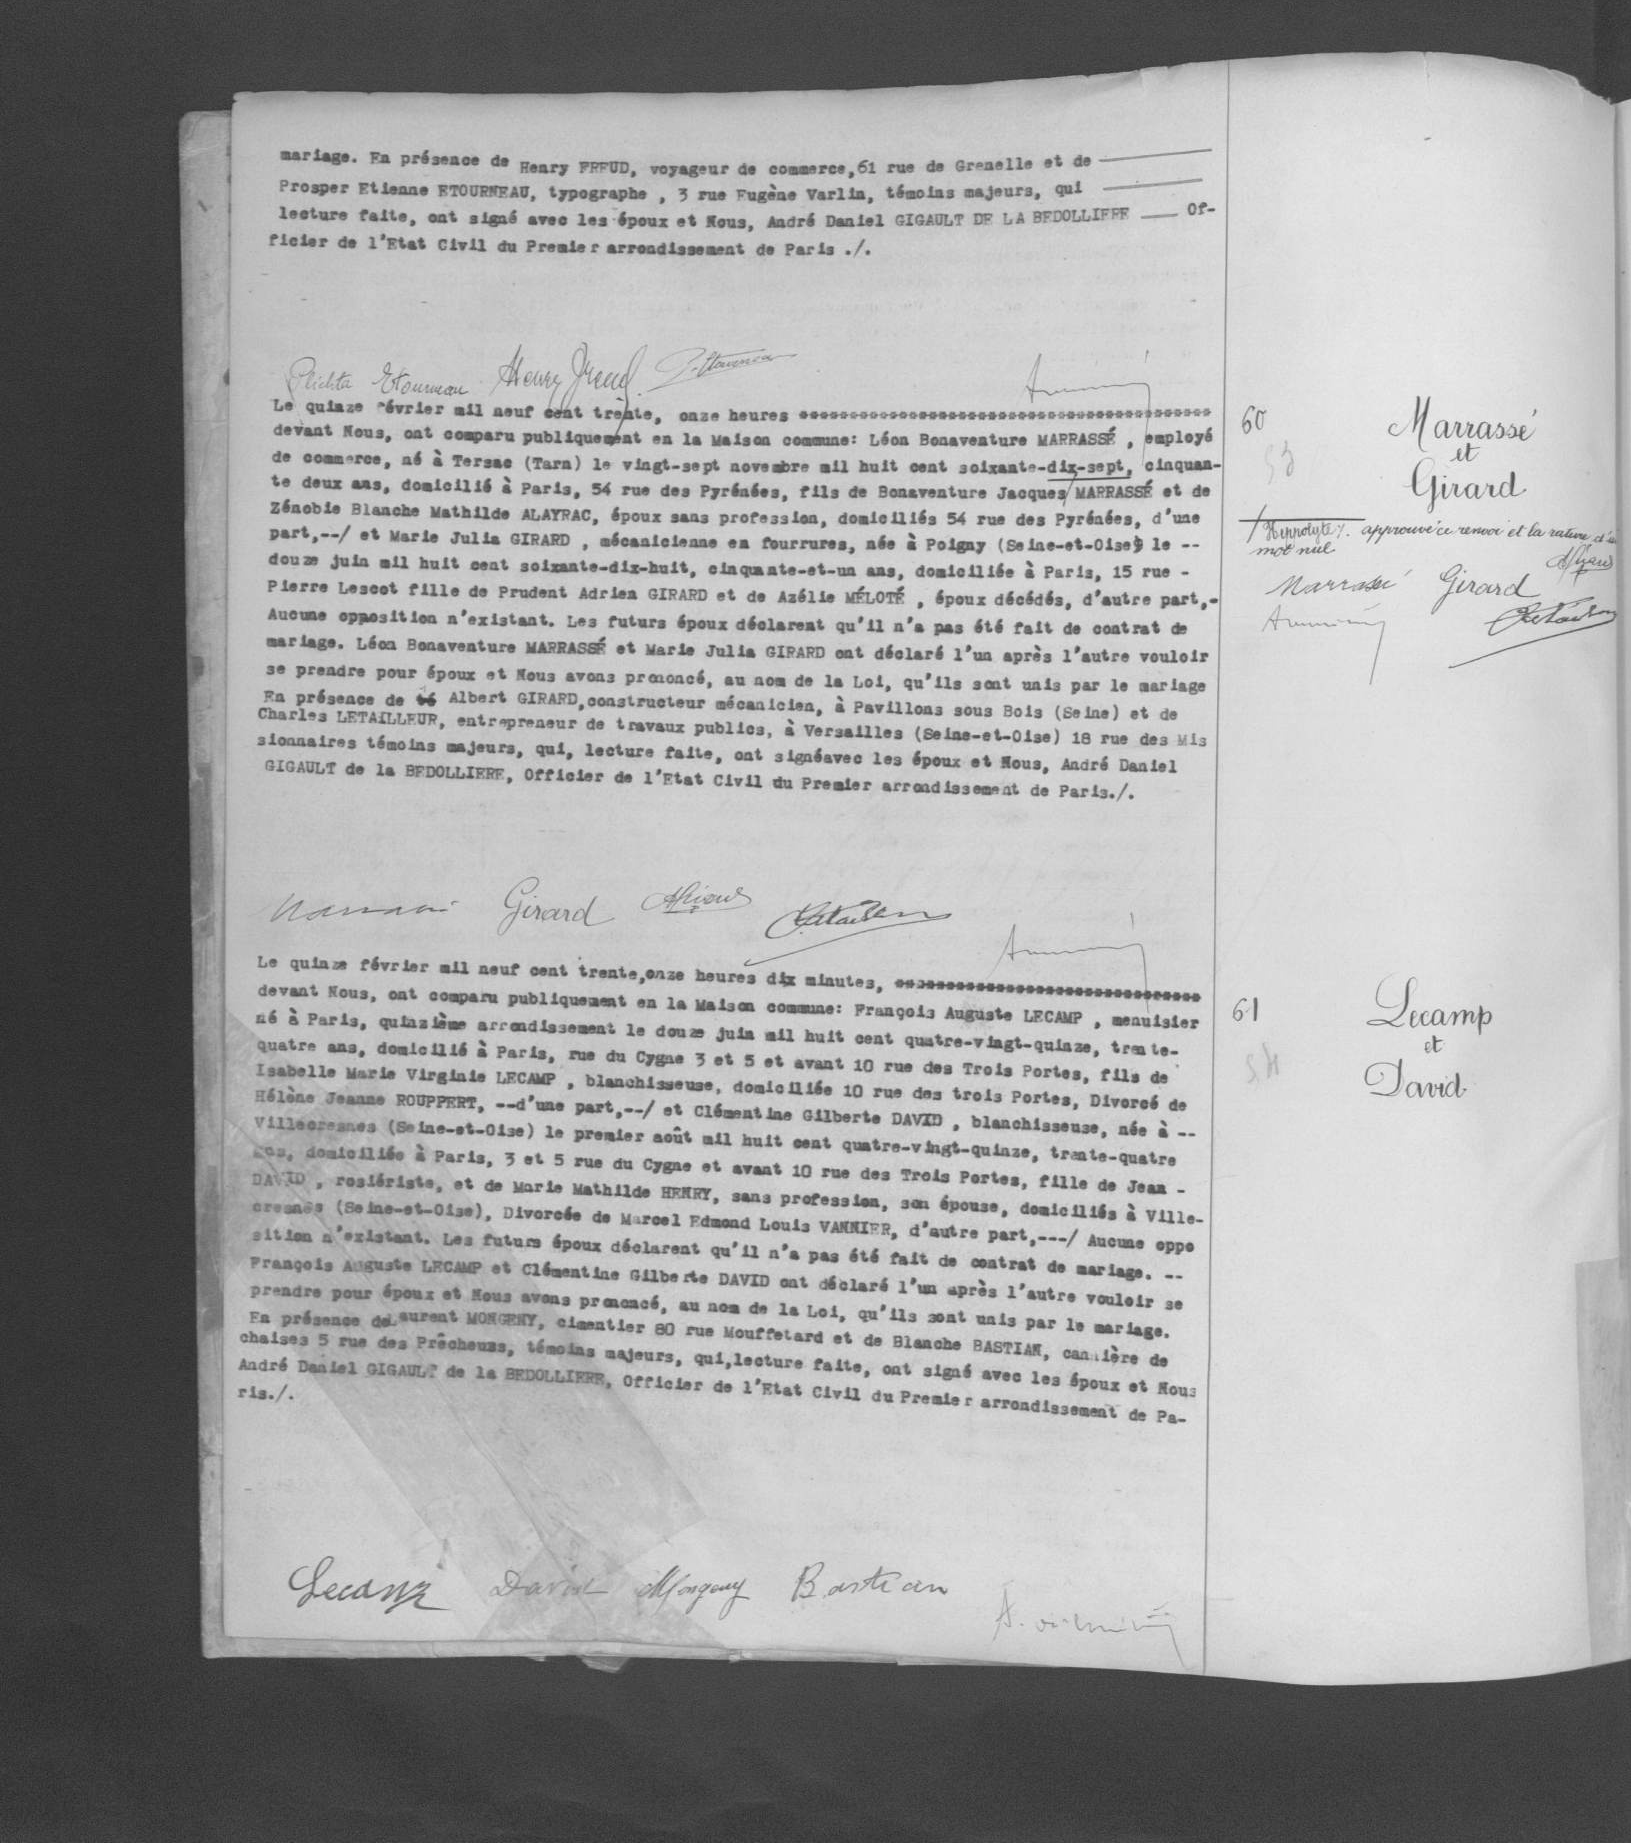mariage. En présence de Henry FREUD, voyageur de commerce, 61 rue de Grenelle et de -
Prosper Etienne ETOURNEAU, typographe, 3 rue Eugène Varlin, témoins majeurs, qui -
lecture faite, ont signé avec les époux et Nous, Anré Daniel GIGAULT DE LA BEDOLLIERE -Of-
ficier de l'Etat Civil du Premier arrondissement de Paris ./.
Le quinze février mil neuf cent trente, onze heures ****
devant Nous, ont comparu publiquement en la Maison commune: Léon Bonaventure MARRASE, empoyé
de commerce, né à Tersac (Tarn) le vingt-sept novembre mil huit cent soixante-dix-sept, cinquan-
te deux ans, domicilié à Paris, 54 rue des Pyrénées, fils de Bonaventure Jacques MARRASE et de
Zénobis Blanche Mathilde ALAYRAC, époux sans profession, domiciliés 54 rue des Pyrénées, d'une
part ,-/ et Marie Julia GIRARD, mécanicienne en fourrures, née à Poigny (Seine-et-Oise) le -
douze juin mil huit cent soixante-dix-huit, cinquante-et-un ans, domiciliée à Paris, 15 rue-
Pierre Lescot fille de Prudent Adrien GIRARD et de Azélie MELOTE, époux décédés, d'autre part ,-
Aucune opposition n'existant. Les futurs époux déclarent qu'il n'a pas été fait de contrat de
mariage. Léon Bonaventure MARRASE et Marie Julia GIRARD ont déclaré l'un après l'autre vouloir
se prendre pour époux et Nous avons prononcé, au nom de la Loi, qu'ils sont unis par le mariage
En présence de Albert GIRARD, constructeur mécanicien, à Pavillons sous Bois (Seine) et de
Charles LETAILLEUR, entrepreneur de travaux publics, à Versailles (Seine-et-Oise) 18 rue des Mis
sionnaires témoins majeurs, qui, lecture faite, ont signé avec les époux et Nous, André Daniel
GIGAULT de la BEDOLLIERE, Officier de l'Etat civil du Premier arrondissement de Paris ./.
Le quinze février mil neuf cent trente, onze heures dix minutes, ****
devant Nous, ont comparu publiquement en la Maison commune: François Auguste LECAMP, menuisier
né à Paris, quinzième arrondissement le douze juin mil huit cent quatre-vingt-quinze, trente-
quatre ans, domicilié à Paris, rue du Cygne 3 et 5 et avant 10 rue des Trois Portes, fils de
Isabelle Marie Virginie LECAMP, blanchisseuse, domiciliée 10 rue des trois Portes, Divorcé de
Hélène Jeanne ROUPPERT, -d'une part, -/ et Clémentine Gilberte DAVID, blanchisseuse, née à -
Villecreanes (Seine-et-Oise) le premier août mil huit cent quatre-vingt-quinze, trante-quatre
ans, domiciliée à Paris, 3 et 4 rue du Cygne et avant 10 rue des Trois Portes, fille de Jean
DAVID, rosiériste, et de Marie Mathilde HENRY, sans profession, son épouse, domiciliés à Ville-
cresnes (Seine-et-Oise), Divorcée de Marcel Edmond Louis VANNIER, d'autre part, -/ Aucune oppo
sition n'existant. Les futurs époux déclarent qu'il n'a pas été fait de contrat de mariage .-
François Auguste LECAMP et Clémentine Gilberte DAVID ont déclaré l'un après l'autre vouloir se
prendre pour époux et Nous avons prononcé, au nom de la Loi, qu'ils sont unis par le mariage.
En présence de Laurent MONGENY, cimentier 80 rue Mouffetard et de Blanche BASTIAN, cannière de
chaises 5 rue des Prêcheurs, témoins majeurs, qui, lecture faite, ont signé avec les époux et Nous
Andre Daniel GIGAULT de la BEDOLLIERE, Officier de l'Etat Civil du Premier arrondissement de Pa-
ris ./.
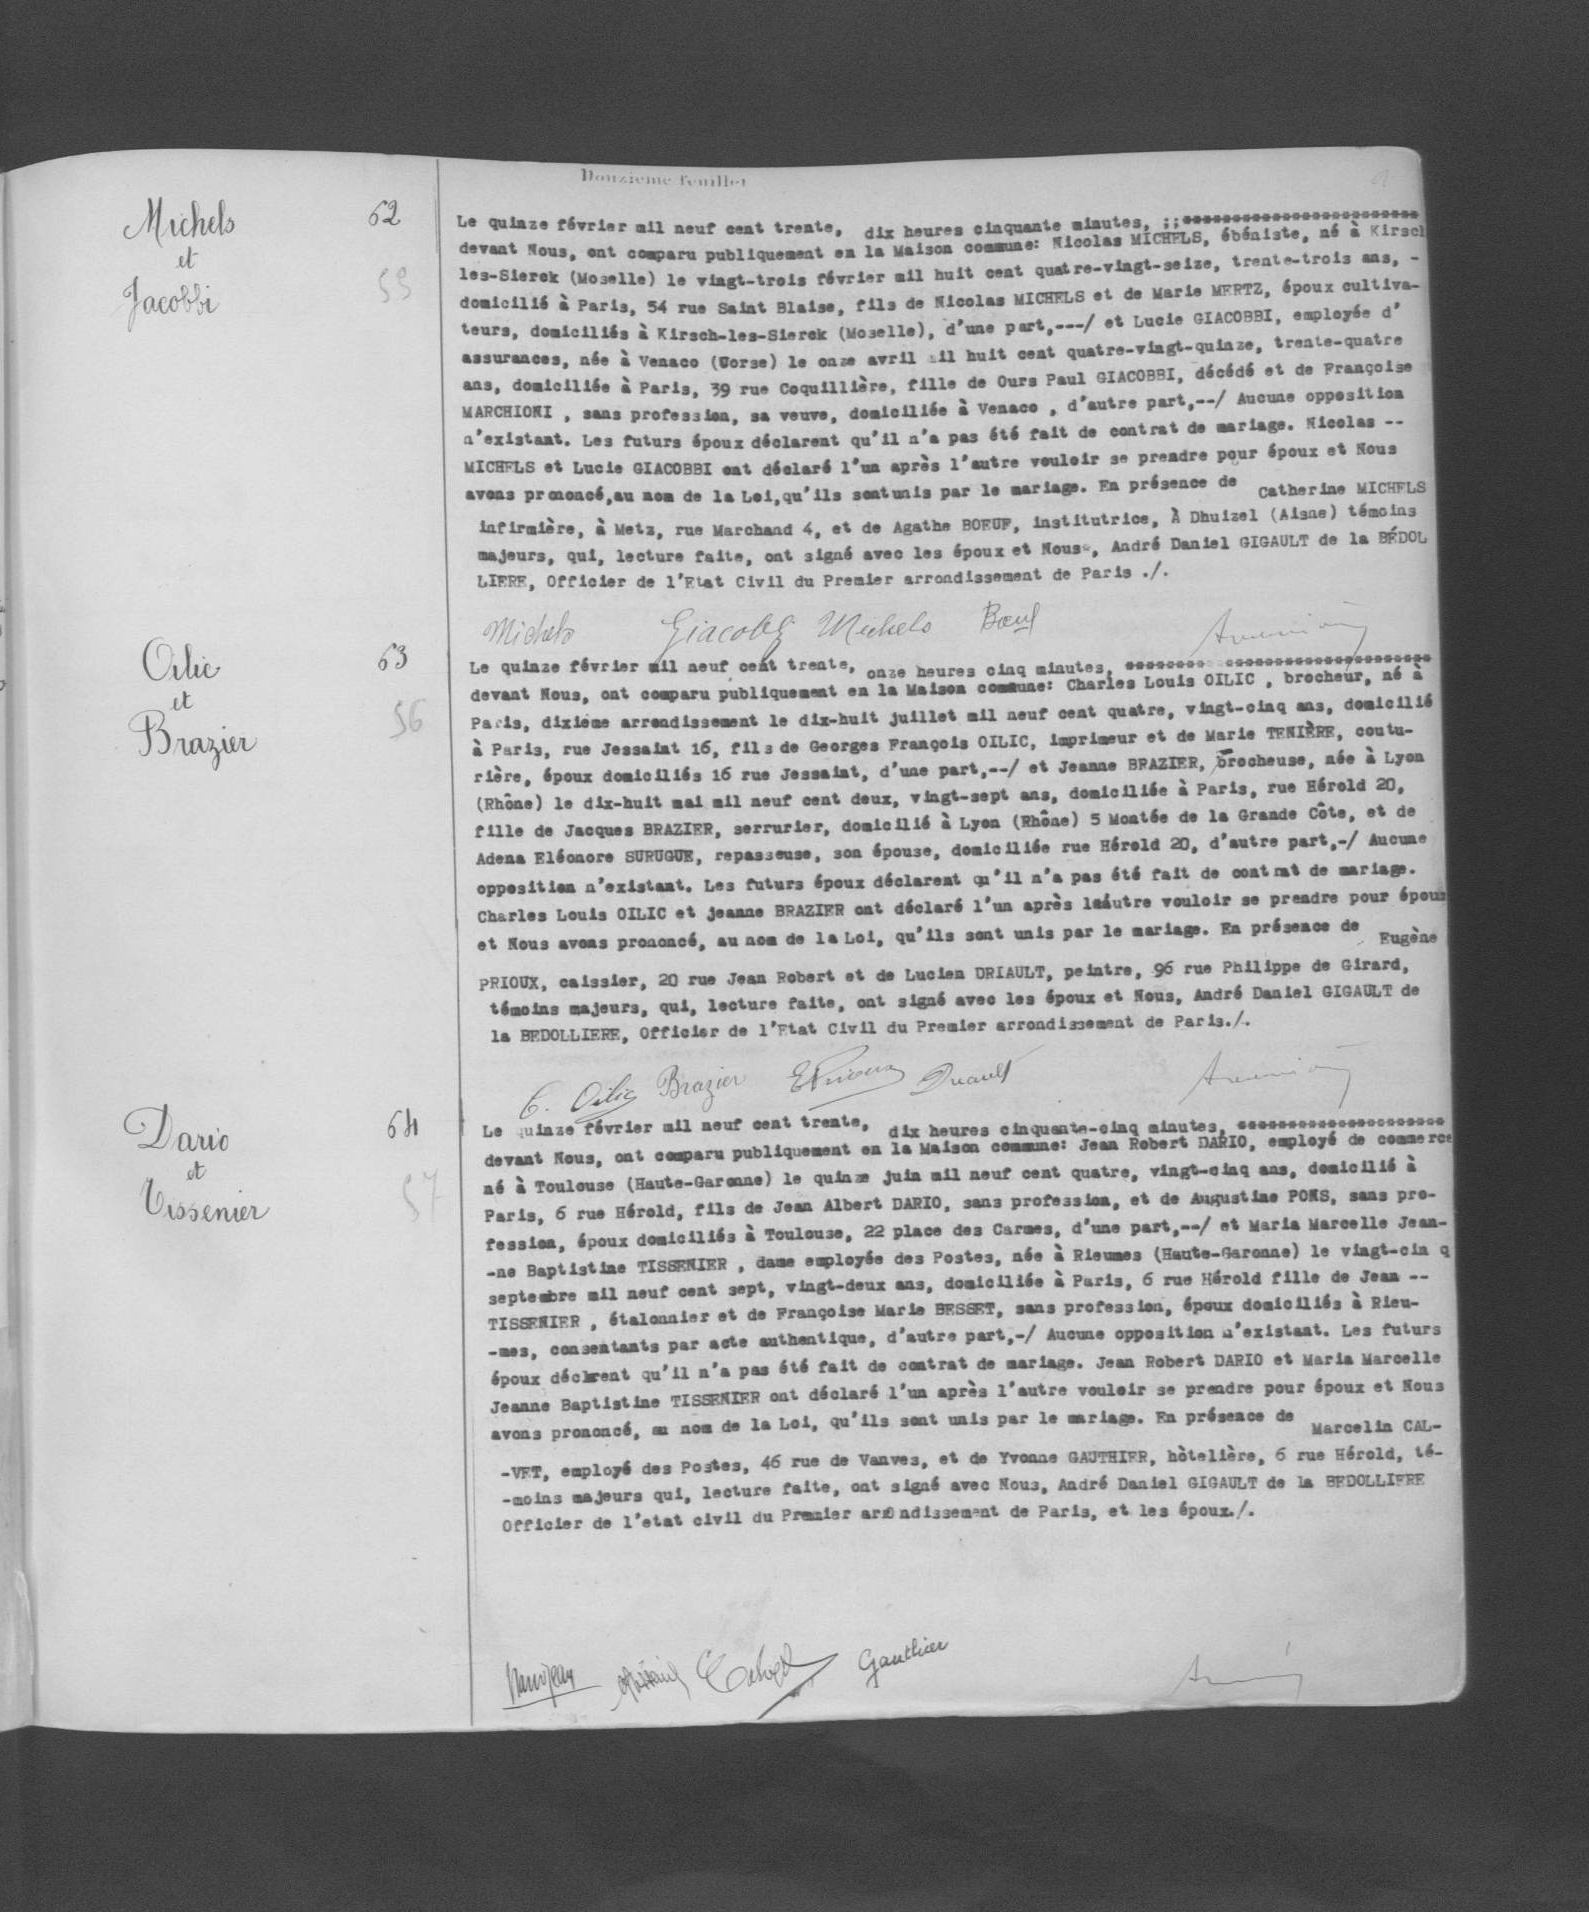Le quinze février mil neuf cent trente, dix heures cinquante minutes, ;; ****
devant Nous, ont comparu publiquement en la Maison commune: Nicolas MICHELS, ébéniste, né à Kirsh
les-Sierek (Moselle) le vingt-trois février mil huit cent quatre-vingt-seize, trente-trois ans,-
domicilié à Paris, 54 rue Saint Blaise, fils de Nicolas MICHELS et de Marie MERTZ, époux cultiva-
teurs, domiciliés à Kirsh-les-Sierek (Moselle) d'une part, -/ et Lucie GIACOBI, employée d'
assurances, née à Venaco (Corse) le onze avril mil huit cent quatre-vingt-quinze, trente-quatre
ans, domiciliée à Paris, 39 rue Coquillière, fille de Ours Paul GIACOBBI, décédé et de Françoise
MARCHIONI, sans profession, sa veuve, domiciliée à Venaco, d'autre part ,-/ Aucune opposition
n'existant. Les futurs époux déclarent qu'il n'a pas été fait de contrat de mariage. Nicolas -
MICHELS et Lucie GIACOBBI ont déclaré l'un après l'autre vouloir se prendree pour époux et Nous
avons prononcé, au nom de la Loi, qu'ils sont unis par le mariage. En présence de Catherine MICHELS
infirmière, à Mets, rue Marchand 4 et de Agathe BOEUF, institutrice, A Dhuizel (Aisne) témoins
majeurs, qui, lecture faite, ont signé avec les époux et Nous, André Daniel GIGAULT de la BEDOL
LIERE, Officier de l'Etat Civil du Premier arrondissement de Paris ./.
Le quinze février mil neuf cent trente, onze heures cinq minutes, ****
devant Nous, ont comparu publiquement en la Maison commune: Charles Louis OILIC, brocheur, né à
Paris, dixième arrondissement le dix-huit juillet mil neuf cent quatre, vingt-cinq ans, domicilié
à Paris, rue Jessaint 16, fils de Georges François OILIC, imprimeur et de Marie TENIERE, coutu-
rière, époux domiciliés 16 rue Jessaint, d'une part, -/ et Jeanne BRAZIER, brocheuse, née à Lyon
(Rhône) le dix-huit mai mil neuf cent deux, vingt-sept ans, domiciliée à Paris, rue Hérold 20,
fille de Jacques BRAZIER, serrurier, domicilié à Lyon (Rhône) 5 Montée de la Grande Côte, et de
Adena Eléonore SURUGUE, repasseuse, son épouse, domiciliée rue Hérold 20, d'autre part ,-. Aucune
opposition n'existant. Les futurs époux déclarent qu'il n'a pas été fait de contrat de mariage.
Charles Louis OILIC et jeanne BRAZIER ont déclaré l'un après l'autre vouloir se prendre pour époux
et Nous avons prononcé, au nom de la Loi, qu'ils sont unis par le mariage. En présence de Eugène
PRIOUX, caissier, 20 rue Jean Robert et de Lucien DRIAULT, peintre, 96 rue Philippe de GIrard,
témoins majeurs, qui, lecture faite, ont signé avec les époux et Nous, André Daniel GIGAULT de
la BEDOLLIERE, Officier de l'Etat civil du Premier arrondissement de Paris ./.
Le quinze février mil neuf cent trente, dix heures cinquante-cinq minutes, ****
devant Nous, ont comparu publiquement en la Maison commune: Jean Robert DARIO, employé de commerce
né à Toulouse (Haute-Garonne) le quinze juin mil neuf cent quatre, vingt-cinq ans, domicilié à
Paris, 6 rue Hérold, fils de Jean Albert DARIO, sans profession, et de Augustine PONS, sans pro-
fession, époux domiciliés à Toulouse, 22 place des Carmes, d'une part ,-/ et Maria Marcelle Jean-
-ne Baptistine TISSENIER, dame employée des Postes, née à Rieumes (Haute-Garonne) le vingt-cinq
septembre mil neuf cent sept, vingt-deux ans, domiciliée à Paris, 6 rue Hérold fille de Jean -
TISSENIER, étalonnier et de Françoise Marie BESSET, sans profession, époux domiciliés à Rieu-
-mes, consentants par acte authentique, d'autre part ,-/ Aucune opposition n'existant. Les futurs
époux, déclarent qu'il n'a pas été fait de contrat de mariage. Jean Robert DARIO et Maria Marcelle
Jeanne Baptistine TISSENIER ont déclaré l'un après l'autre vouloir se prendre pour époux et Nous
avons prononcé, au nom de la Loi, qu'ils sont unis par le mariage. En présence de Marcelin CAL-
-VET, employé des Postes, 46 rue de Vanves, et de Yvonne GAUTHIER, hôtelière, 6 rue Hérold, té-
-moins majeurs qui, lecture faite, ont signé avec Nous, André Daniel GIGAULT de la BEDOLLIERE
Officier de l'état civil du Premier arrondissement de Paris, et les époux ./.